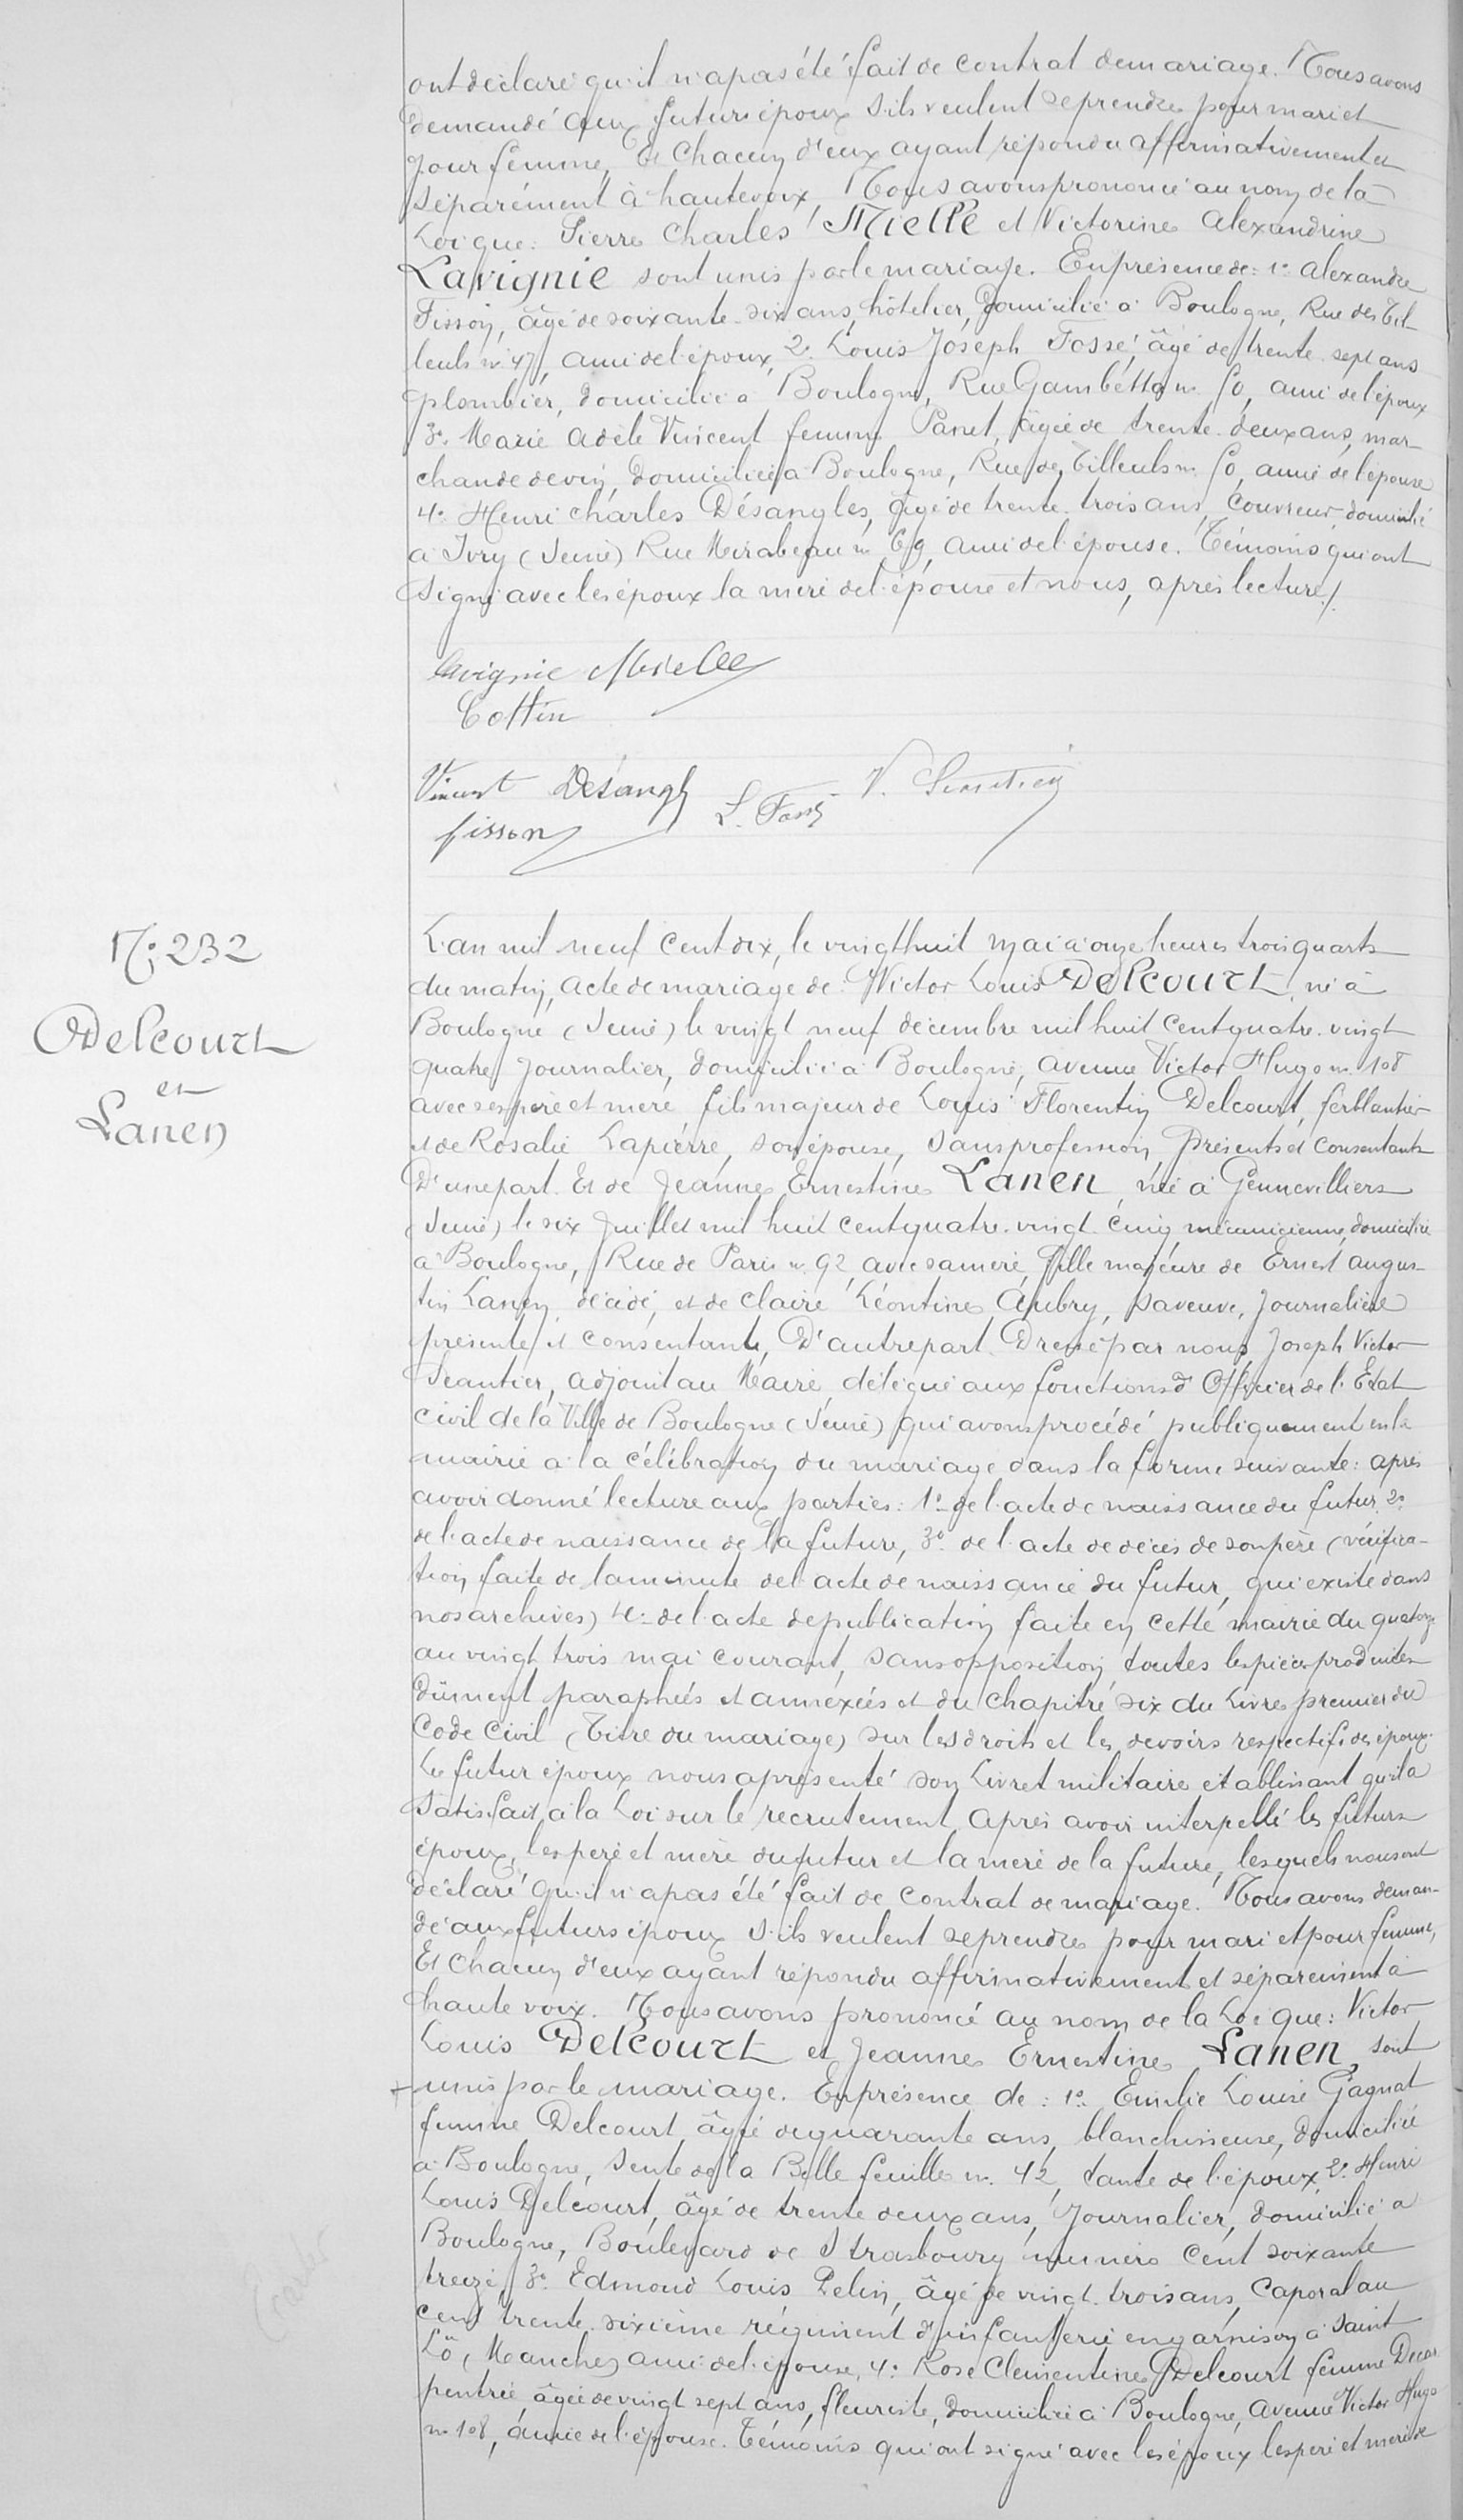ont déclaré qu'il n'a pas été fait de contrat de mariage. Nous avons
demandé aux futurs époux s'ils veulent se prendre pour mari et
pour femme Chacun d'eux ayant répondu affirmativement et
séparément à hautevoix, Nous avons prononcé au nom de la
Loi que Pierre Charles Mielle et Victorine Alexandrine
Lavignie sont unis par le mariage. En présence de 1° Alexandre
Fisson, âgé de soixante-dix ans, hôtelier, domicilié à Boulogne, Rue de Til-
leuls n°47, ami de l'époux 2°Louis Joseph Fosse âgè de trente-sept ans
plombier, domicilié à Boulogne, Rue Gambetta n°10, ami de l'époux
3° Marie Adèle Vincent femme Panel, âgée de trente-deux ans, mar-
chande de vin, domiciliée à Boulogne, Rue de Tilleuls n°1°, amie de l'épouse
4° Henri Charle Désangles, âgé de trente-trois ans, couvreur, domicilié
à Ivry (Seine) Rue Mirabeau N 69, ami de l'épouse. Témoins qui ont
signé avec les époux, la mère de l'épouse et nous, après lecture./.
N° 232
Delcourt
et
Laner
L'an mil neuf cent dix, le vingthuit mai à onze heures trois quarts-
du matin, acte de mariage de Victor Louis Delcourt, né à
Boulogne (Seine) le vingt neuf décembre mil huit cent quatre-vingt
quatre journalier, domicilié à Boulogne, avenue Victor Hugo n°108
avec ses père et mère, fils majeur de Louis Florentin Delcourt, ferblantier
et de Rosalie Lapierre, son épouse, sans profession, présents et consentants,
D'une part. Et de Jeanne Ernestine Lanen, née à Gennevilliers
(Seine) le six Juillet mil huit cent quatre-vingt cinq mécanicienne, domiciliée
à Boulogne, Rue de Paris n°92, avec sa mère, fille majeure de Ernest Augus-
tin Laney, décédé, et de Claire Léontine Apibry, sa veuve, journalère
présente et consentante, D'autre part. Dressé par nous, Joseph Victor
Seantier, adjoint au Maire délégué aux fonctions, d'Officier de l'Etat
Civil de la Ville de Boulogne (Seine) qui avons procédé publiquement en la
mairie à la célébration, du mariage dans la forme suivante: après
avoir donné lecture aux partie: 1° de l'acte de naissance du futur 2°
de l'acte de naissance de la future, 3° de l'acte de décès de son père (vérifica-
tion faite de la minute de l'acte de naissance du futur, qui existe dans
nos archives) 4° de l'acte de publication faite en cette mairie du quatorze
au vingt trois mai courant, sans opposition, toutes les pièces produites
dûment paraphées et annexées et du chapitre six du libre premier du
Code Civil (Titre du mariage) sur les droits et les devoirs respectif des époux
Le futur époux nous a,présenté son livret militaire établissant qu'il a
satisfait à la loi sur le recrutement après avoir interpellé les futurs
époux, les père et mère du futur et la mère de la future, lesquels nous ont
déclaré qu'il n'a pas été fait de contrat de mariage. Nous avons deman-
dé aux futurs époux s'ils veulent seprendre pour mari et pour femme,
Et chacun d'eux ayant répondu affirmativement et séparement à
haute voix. Nous avons prononcé au nom de la Loi que: Victor
Louis Delcourt et Jeanne Ernestine Lanen sont
unis par le mariage. En présence de 1° Emilie Louise Gagnat
femine Delcourt âgé de quarante ans, blanchisseuse, domiciliée
à Boulogne, Seule de la Belle feuille n 12, tant de l'époux 2° Henri
Louis Delcourt, âgé de trente deux ans, Journalier, domicilié a
Boulogne, Boulevard de Strasbourg numéro cent soixante
treize 3° Edmond Louis Pelin, âgé de vingt-trois ans, Caporal au
cent trente sixième régiment d'infanterie engarnison à Saint
Lô, Manche, ami de l'épouse, 4° Rose Clémentine Delcourt femme Decos
peintre, âgèe de vingt sept ans, fleuriste, domiciliée à Boulogne, Avenue Victor Hugo
n 108, amie de l'épouse. Témoins qui ont signé avec les époux les père et mère de
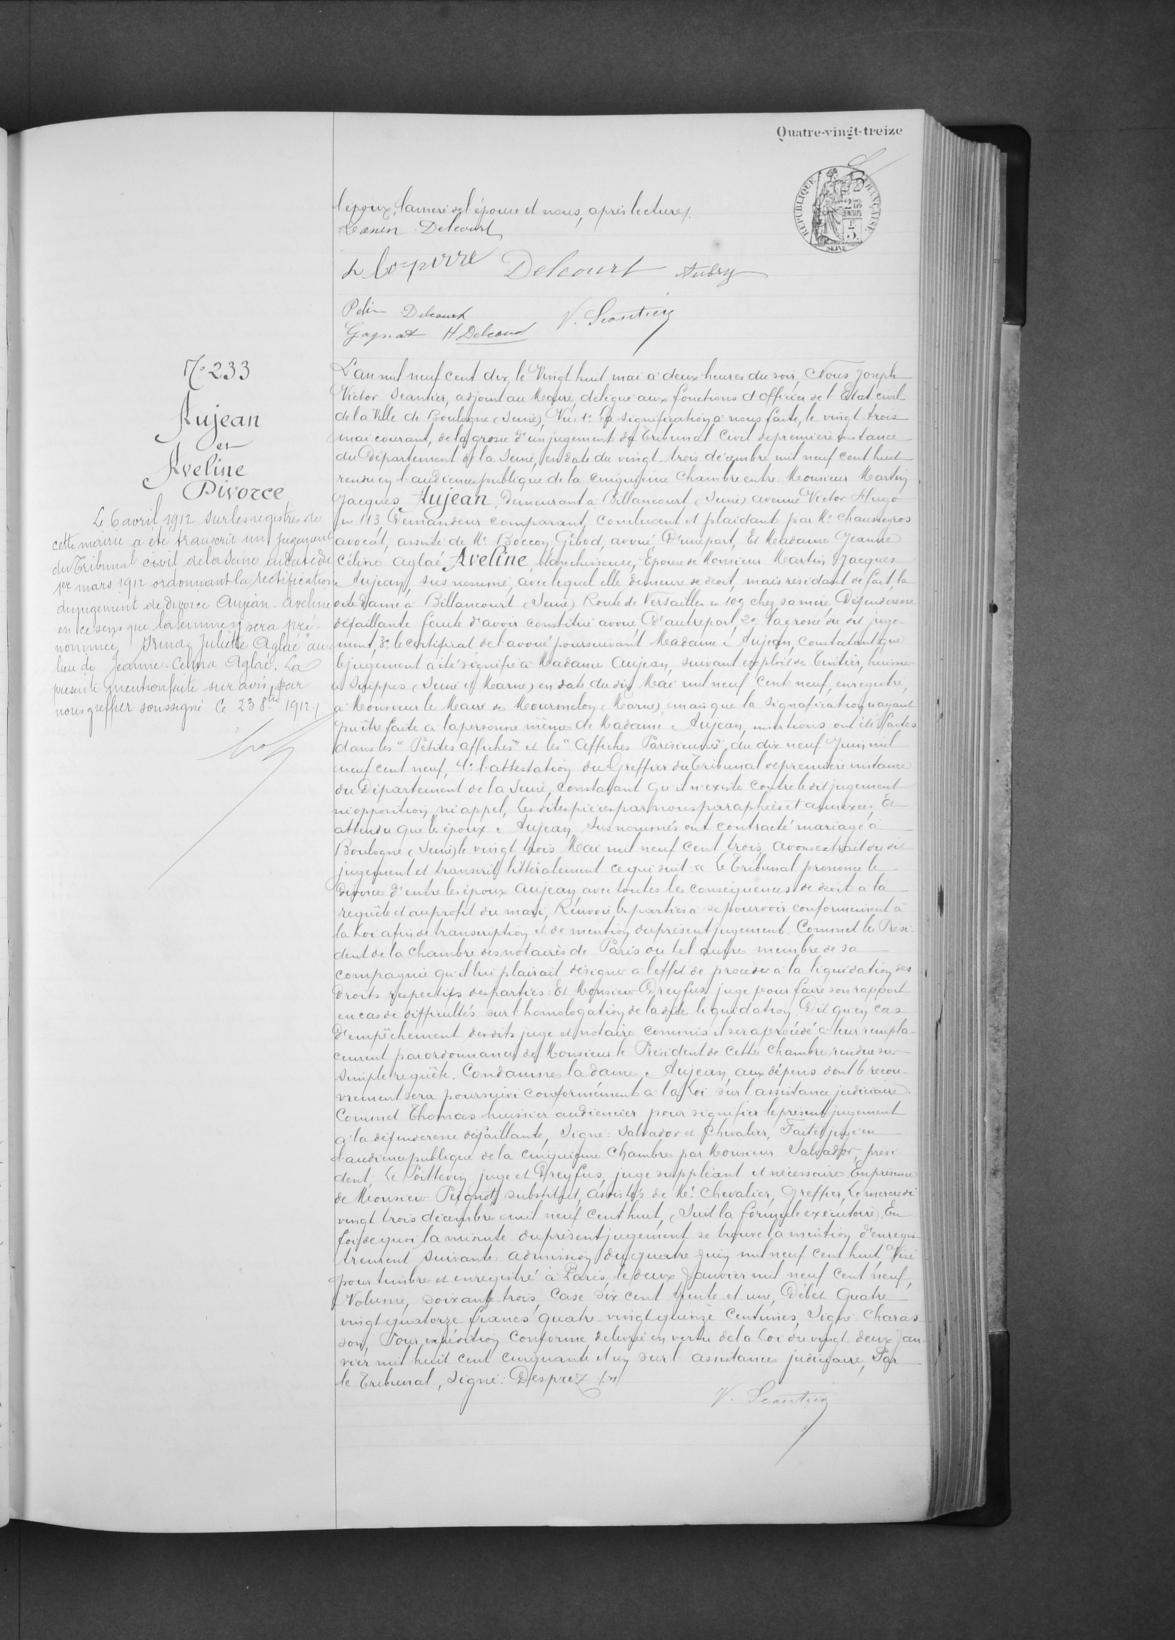l'époux, la mère de l'épouse et nous, après lecture./.
Lanen Deleourt
N°233
Aujeau
et
Aveline
Divorce
L'an mil neuf cent dix, le Vingt huit mai à deux heures du soir, Nous Joseph
Victor Seantier, adjoint au Maire, délégué aux fonctions d'Officier de l'Etat civil
de la Ville de Boulogne (Seine) Vu: 1°: La signification à nous faite, le vingt-trois
mai courant, de la grosse d'un jugement de Tribunal Civil de première instance
du Département de la Seine, en date du vingt-trois décembre mil neuf cent huit
rendu en l'audience publique de la cinquième chambre entre: Monsieur Matin
Jacques Aujean, demeurant à Billancourt (Seine) avenue Victor Hugo
au 113, Demandeur comparant concluant et plaidant par M Chaussegros
avocat, assisté de Me Boccon, Gibod, avoué D'une part, et Madame (Jeanne)
Céline Aglaé Aveline, blanchisseuse, Epouse de Monsieur Martin Jacques
Aujean, sus nommé, avec lequel elle demeure de droit, mais résidant de fait la
dite dame à Billancourt (Seine) Route de Versaille en 10° chez sa mère, Défenderesse
défaillante faute d'avoir constitué avoué D'autre part, 2° la grosse du dit juge-
ment, 3° certificat del'avoué poursuivant Madame Aujean, constatant que
le jugement a été signifié à Madame Aujean, suivant exploit de Tenitier, huissier
à Dieppes (Seine et Marne) en date du six Mai mil neuf cent neuf, enregistré,
à Monsieur le Maire de Mourmeloy (Marne) mais que la signification n'ayant
pu être faite à la personne même de Madame Aujean, insertions ont été faites
dans les "Petites Affiches" et les "Affiches Parisiennes" des dix neuf Juin mil
neuf cent neuf, 4° l'attestation du Greffier du Tribunal depremière instance
du Département de la Seine, constatant qu'il n'existe contre le dit jugement
ni opposition ni appel, les dites pièces par nous paraphées et annexées Et
attendu que les époux Aujean susnommés ont contracté mariage à
Boulogne (Seine) le vingt trois Mai mil neuf cent trois, avons extrait du dit
jugement et transcrit littéralement ce qui suit:" Le Tribunal prononce le
divorce d'entre les époux Aujeau, avec toutes les conséquences de droit à la
requête et auprofit du mari, Renvoi les parties à sepourvoir conformément à
la Loi afin de transcription et de mention duprésent jugement Commet le Prési-
dent de la Chambre des notaires de Paris ou tel autre membre de sa
compagnie qu'il lui plairait désigner à l'effet de procéder à la liquidation des
droits respectifs des parties: Et Monsieur Dreyfus juge pour faire son rapport
en cas de difficultés sur l'homologation de la dite liquidation. Dit qu'en cas
d'empêchement des dits juge et notaire commis il seraprocédé à leur rempla-
cement parordonnance de Monsieur le Président de cette chambre rendre sur
simple requête. Condamne la dame Aujeau, aux dépens dont le recou
vrement sera poursuivi conformément à la Loi sur l'assistance judiciaire.
Commet Thomas huissier audiencier pour signifier le présent jugement
à la défenderesse défaillante, signé: Salvador et Chevalier Faite jusqu'en -
d'audience publique de la cinquième Chambre par Monsieur Salvador, prési
dent, Le Poillevin juge et Dreyfus, juge suppléant et nécessaire. Enprésence
de Monsieur Peignot substitut, assistés de Me Chevalier, Greffier, Le mercredi
vingt trois décembre avril neuf cent huit, suit la formule exécutoire. En
Loi de quoi la minute du présent jugement se trouve la mention d'enregis-
trement suivante: admission du quatre juin mil neuf cent neuf,
Volume, soixante-trois, case dix cent trente-et-un, Débet Quatre
vingt-quatorze francs quatre-vingt quinze centuries, Signé: Charas
son, Pour expédition, Condorme délivrée en vertu de la Loi, du vingt deux Jan
vier mil huit cent cinquante et un sur l'assistance judiciaire, Par
le Tribunal, Signé: Desprez./.